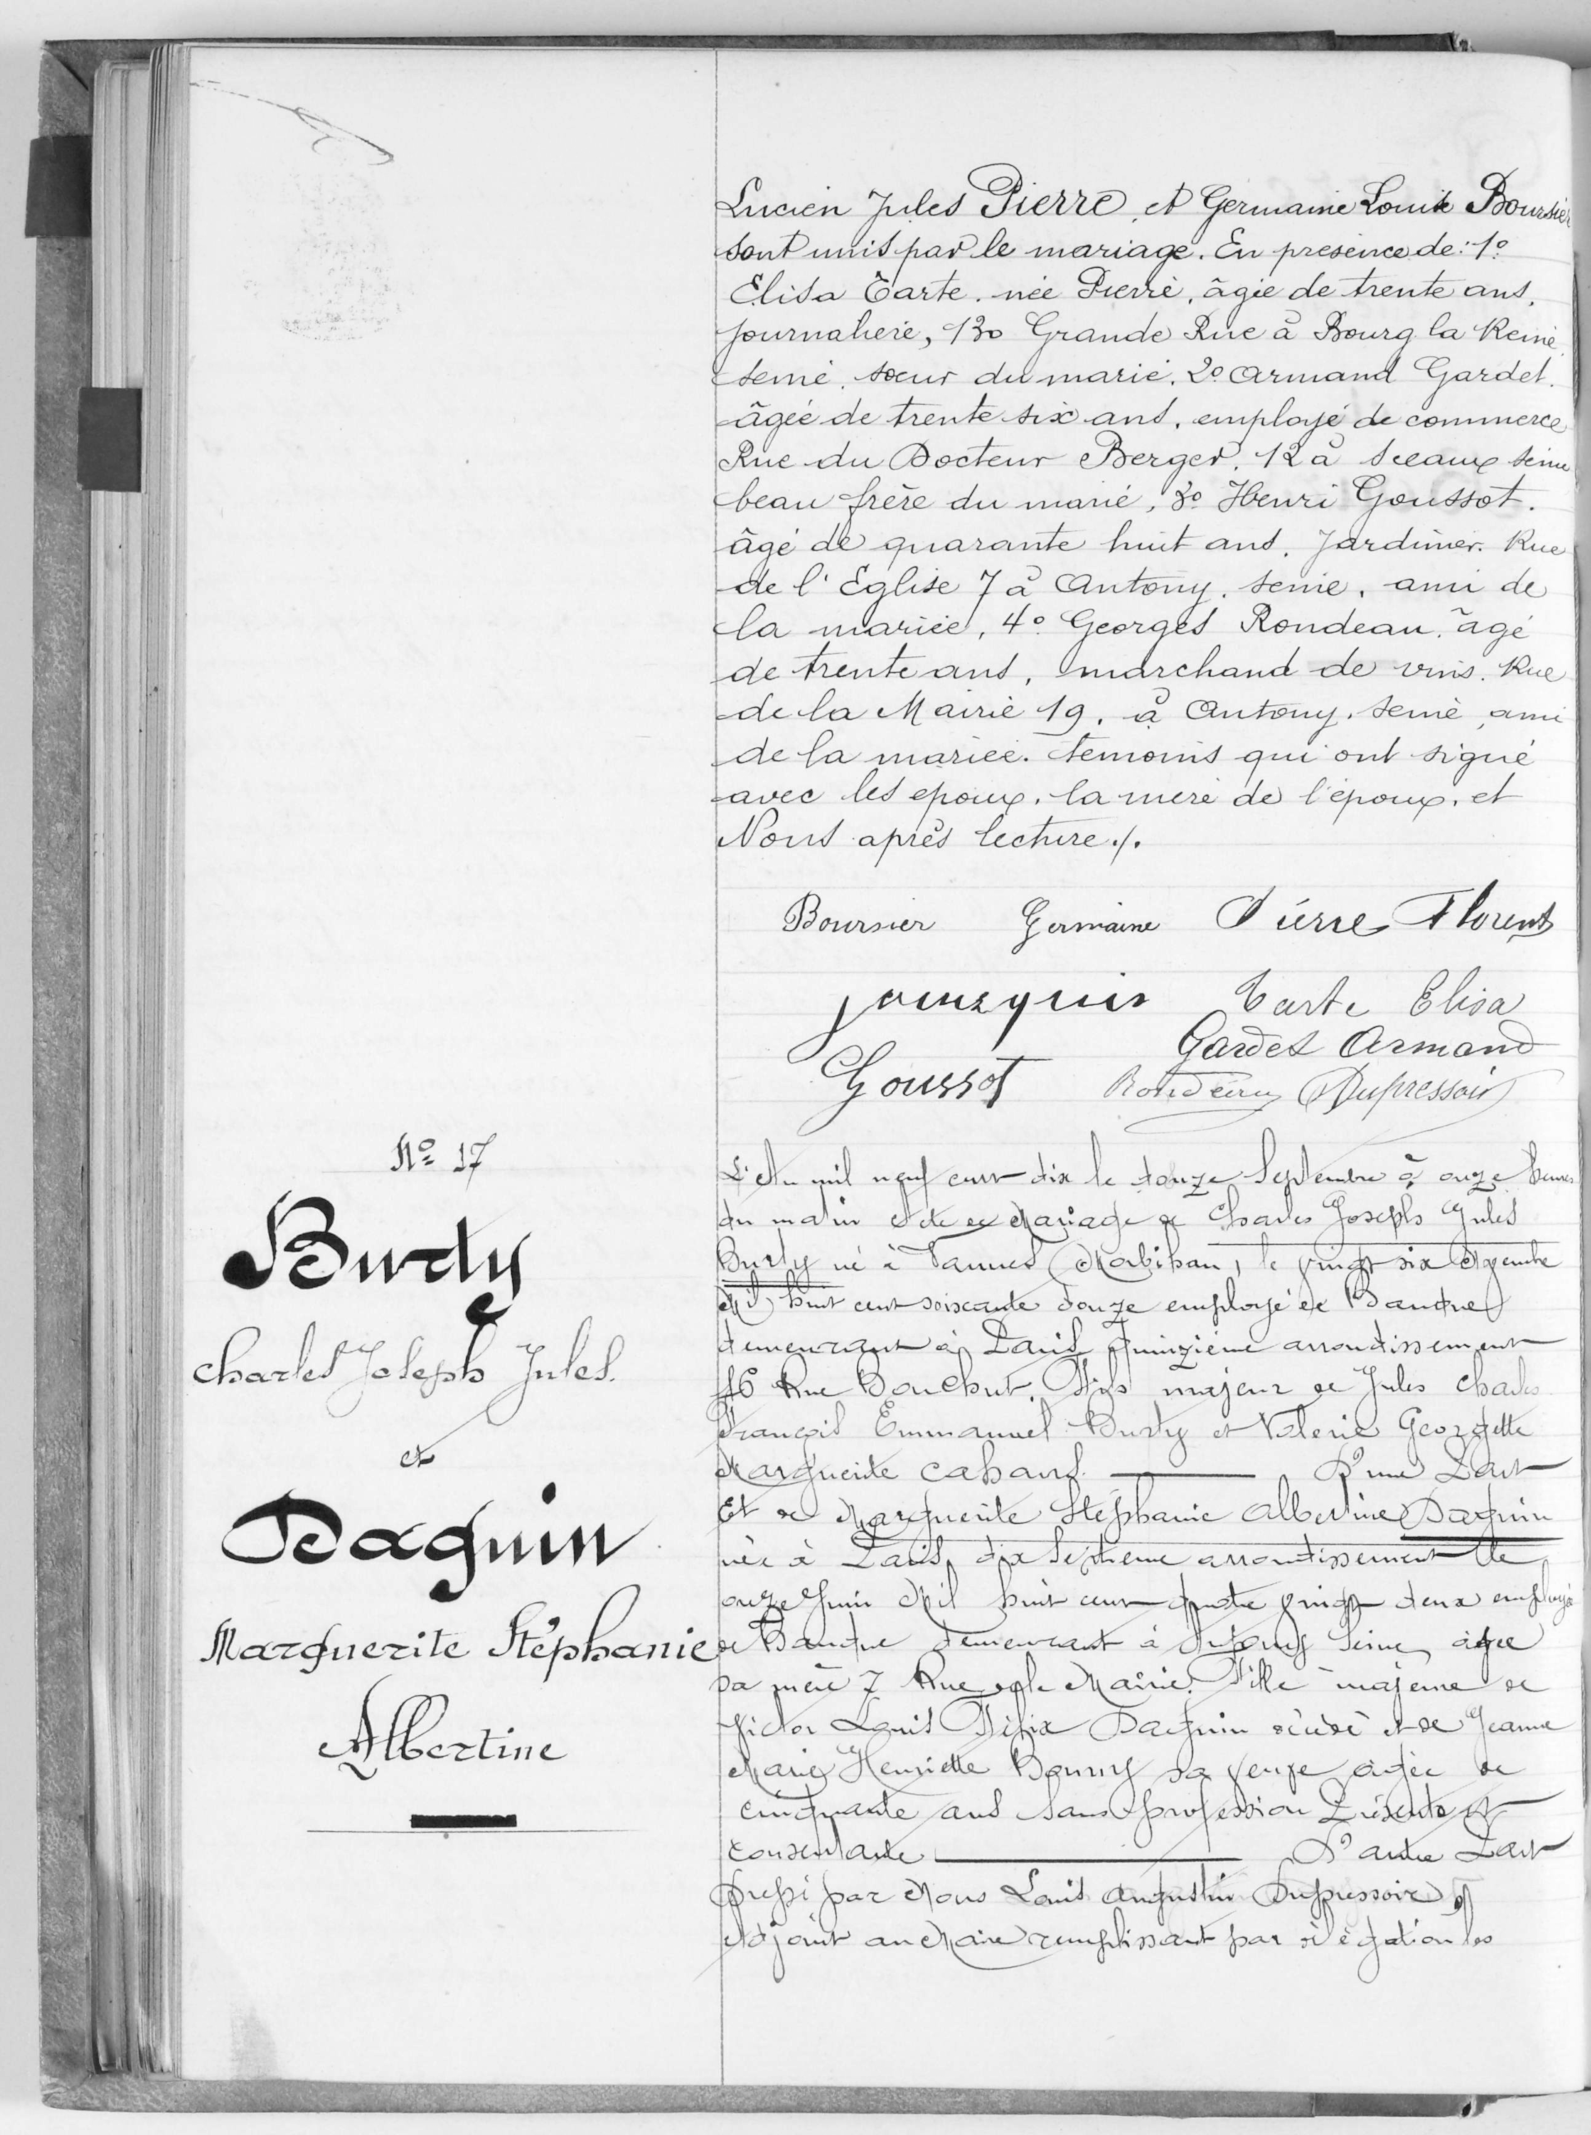Lucien Jules Pierre et Germaine Louise Boursier
sont unis par le mariage. En présence de 1°
Elisa Tarte, née Pierre, âgée de trente ans,
journalière, 130 Grande Rue à Bourg la Reine
Seine, soeur du marié, 2° Armand Gardet
âgée de trente six ans, employé de commerce
Rue du Docteur Berger, 12 à Sceaux Seine
beau frère du marié, 3° Henri Goussot,
âgé de quarante huit ans, jardinier, Rue
de l'Eglise 7 à Antony, seine, ami de
la mariée, 4° Georges Rondeau, âgé
de trente ans, marchand de vins, Rue
de la Mairie 19, à Antony, seine, ami
de la mariée, témoins qui ont signé
avec les époux, la mère de l'époux, et
Nous après lecture ./.
N°17
Burly
Charles Joseph Jules
et
Dagnin
Marguerite Stéphanie
Albertine
L'An mil neuf cent dix le douze Septembre à onze heures
du matin acte de mariage de Charles Joseph Jules
Burty né à Vannes (Morbihan) le vingt six Novembre
mil huit cent soixante douze employée en Banque
demeurant à Paris quinzième arrondissement
16 Rue Bouchut, fils majeur de Jules Charles
François Emmanuel Burty et Valerie Georgette
Marguerite Cahairs. -D'une part
Et de Marguerite Stéphanie Albertine Daguin
née à Paris, dix Septième arrondissement le
onze juin mil huit cent quatre vingt deux employée
de Banque demeurant à Antony Seine, avec
sa mère 7 Rue de la Mairie, fille majeure de
Victor Louis Félix Daguin décédé et de Jeanne
Marie Henriette Bourny sa veuve agée de
cinquante ans sans profession présente et
consentante -D'autre Part
Dressé par Nous Louis Augustine Dupressoire,
adjoint au Maire remplissant par délégation les
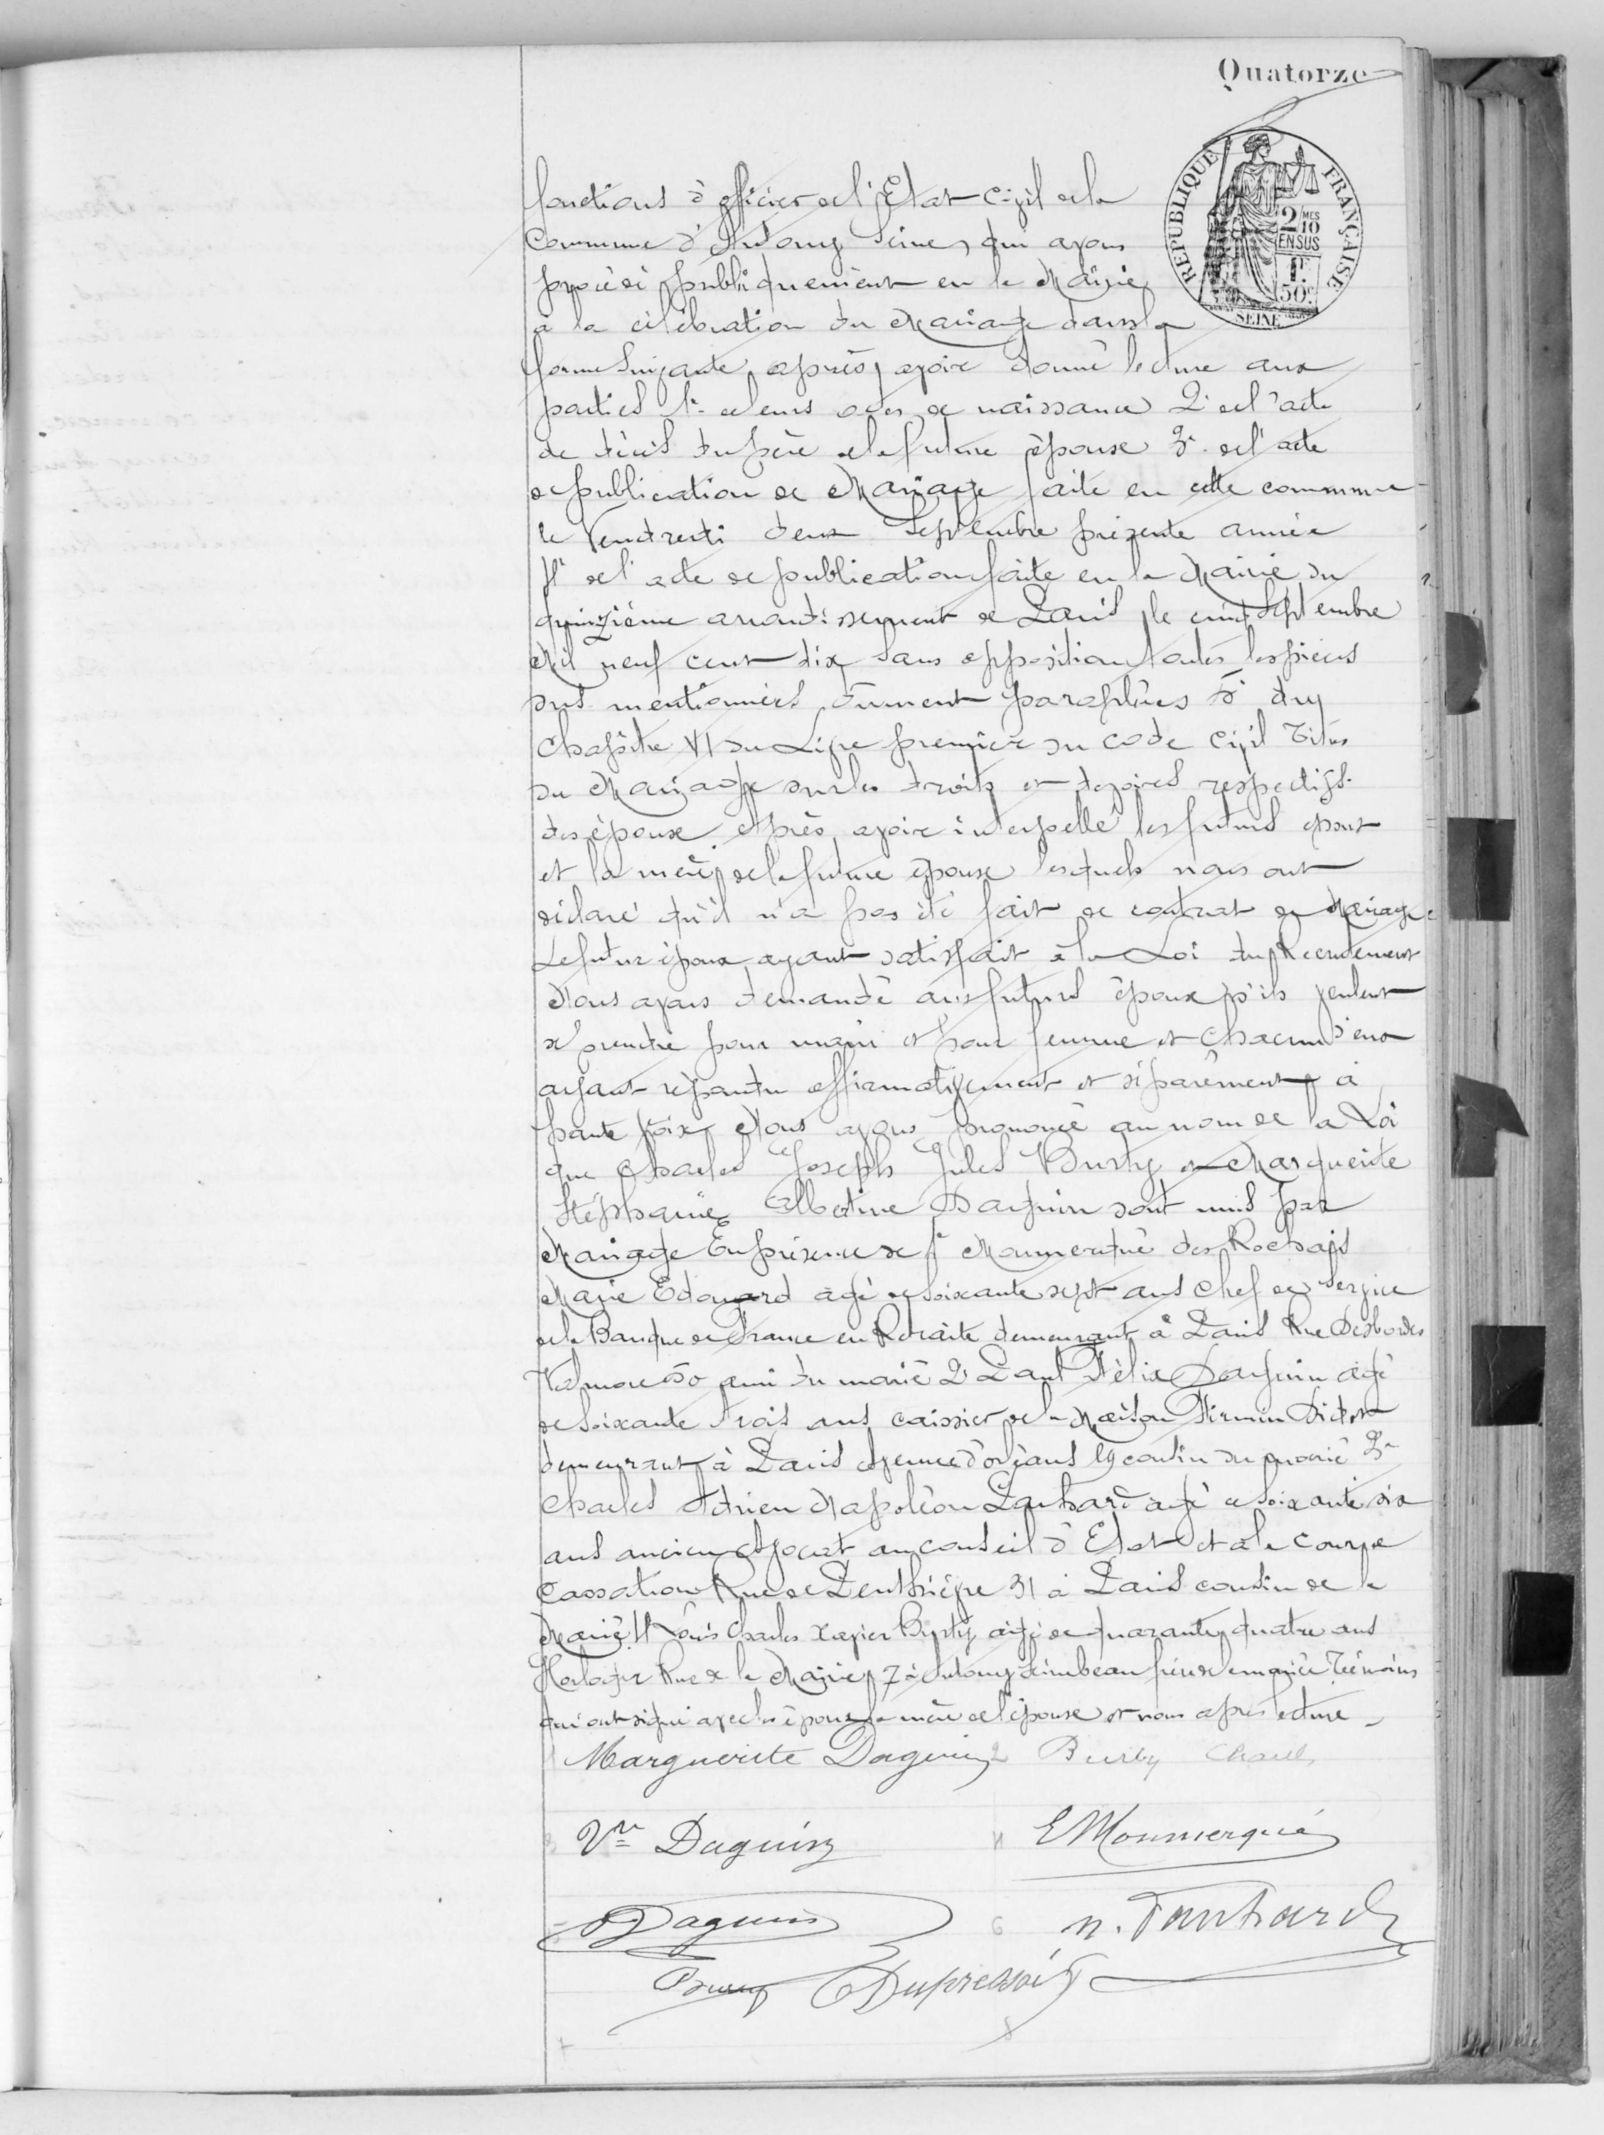fonctions à officier de l'Etat civil à la
commune d'Antony Seine qui avons
procédé publiquement en la Mairie
à la célébration du Mariage dans la
forme suivante après avoir donné lecture aux
parties 1° leurs actes de naissance 2° de l'acte
de décès du père de la future épouse 3° de l'acte
de publication de mariage faite en cette commune
le Vendredi deux septembre présente année
4° l'acte de publication faite en la Mairie du
quinzième arrondissement de Paris, le cinq septembre
mil neuf cent dix sans opposition toutes les pièces
sus mentionnés dument paraphées & du
chapitre VI du Livre premier du Code Civil Titre
du Mariage sur les droits et devoirs respectifs
des époux. Après avoir interpellé les futurs époux
et la mère de la future épouse lesquels nous ont
déclaré qu'il n'a pas été fait de contrat de Mariage.
Le futur époux ayant satisfait à la Loi du Recrutement
Nous avons demandé aux futurs époux s'ils veulent
se prendre pour mari et pour femme et chacun d'eux
ayant répondu affirmativement et séparément à
haute voix nous avons prononcé au nom de la Loi
que Charles Joseph Jules Burty et Marguerite
Stéphanie Albertine Daguin sont unis par le
Mariage En présence de 1° Moumerotué des Rochais
Maire Edouard agé de soixante sept ans chef de service
à la Banque de France en Retraite demeurant à Paris Rue Desbordes
Valmore ami du marié 2 Paul Félix Daguin agé
de soixante trois ans caissier de la maison Firmin Victor
demeurant à Paris Avenue d'orléans 19 cousin du marié 3°
Charles Adrien Napoléon Larhard âgé de soixante six
ans ancien avocat au conseil d'Etat et à la cour de
Cassation Rue de Penthièvre 31 à Paris cousin de la
mariée 4 Louis Charles Xavier Burty agé de quarante quatre ans
Horloger Rue de la Mairie 7 à Antony Seine beau frère du marié témoins
qui ont signé avec les époux la mère de l'épouse et nous après lecture